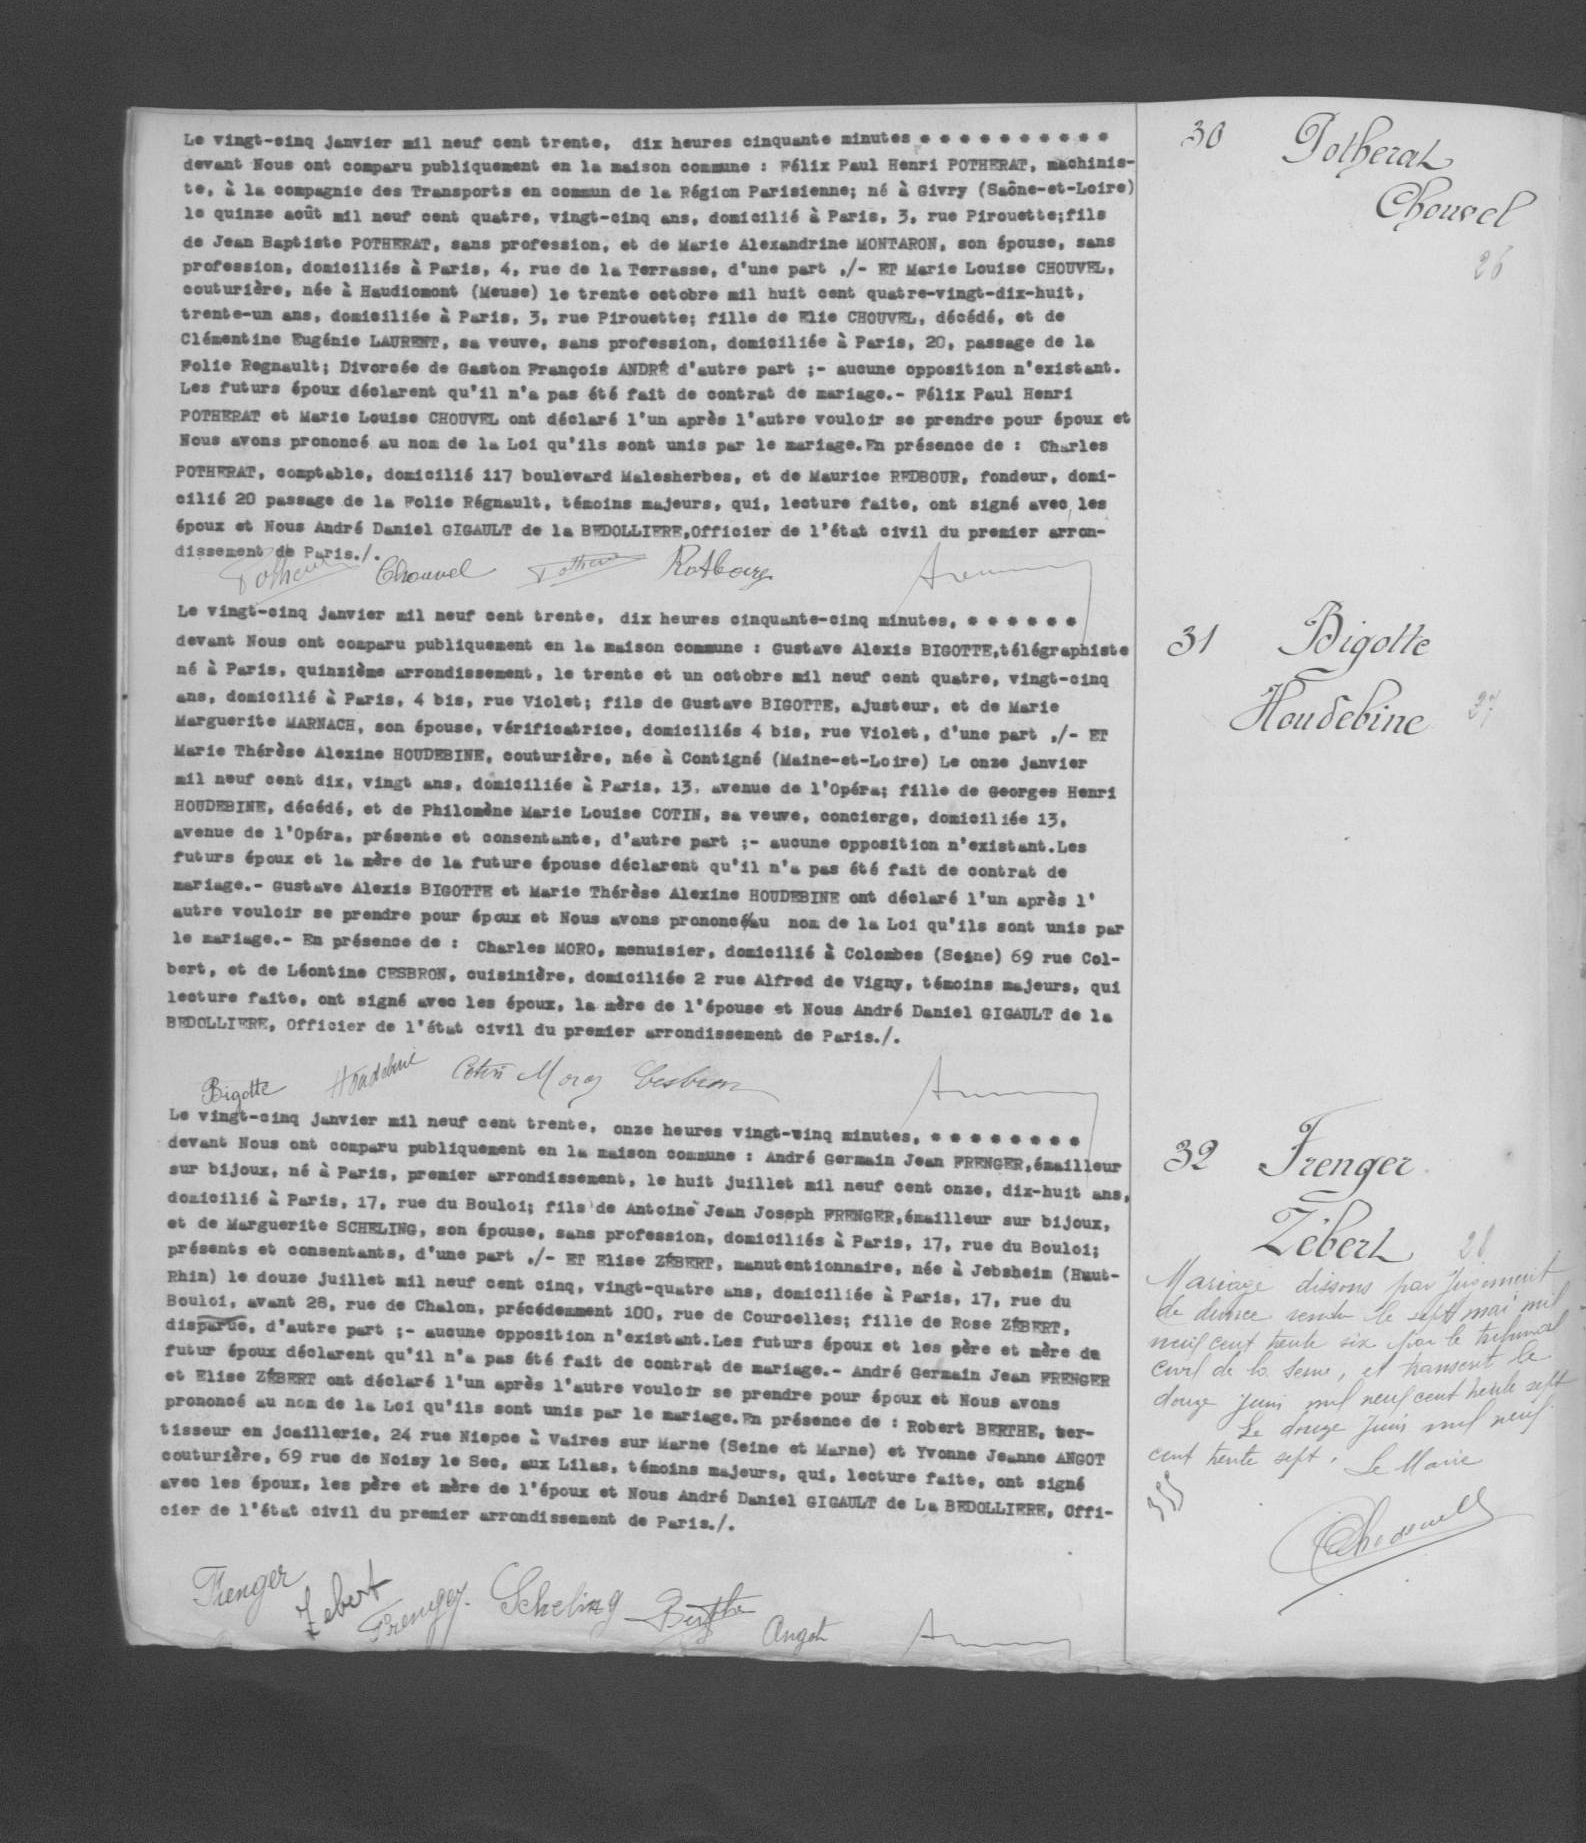Le vingt-cinq janvier mil neuf cent trente, dix heures cinquante minutes ****
devant Nous ont comparu publiquement en la maison commune: Félix Paul Henri POTHERAT, machinis-
te, à la compagnie des Transports en commun de la Région Parisienne; né à Givry (Saône-et-Loire)
le quinze août mil neuf cent quatre, vingt-cinq ans, domicilié à Paris, 3, rue Pirouette; fils
de Jean Baptiste POTHERAT, sans profession, et de Marie Alexandrine MONTARON, son épouse, sans
profession, domiciliés à Paris, 4, rue de la Terrasse, d'une part ./-ET Marie Louise CHOUVEL,
couturière, née à Hauiomont (Meuse) le trente octobre mil huit cent quatre-vingt-dix-huit,
trente-un ans, domiciliée à Paris, 3, rue Pirouette; fille de Elie CHOUVEL, décédé, et de
Clémentine Eugénie LAURENT, sa veuve, sans profession, domiciliée à Paris, 20, passage de la
Folie Regnault; Divorcée de Gaston François ANDRE d'autre part ;-aucune opposition n'existant.
Les futurs époux déclarent qu'il n'a pas été fait de contrat de mariage.-Félix Paul Henri
POTHERAT et Marie Louise CHOUVEL ont déclaré l'un après l'autre vouloir se prendre pour époux et
Nous avons prononcé au nom de la Loi qu'ils sont unis par le mariage. En présence de: Charles
POTHERAT, comptable, domicilié 117 boulevard Malesherbes, et de Maurice REDBOUR, fondeur, domi-
cilié 20 passage de la Folie Régnault, témoins majeurs, qui, lecture faite, ont signé avec les
époux et Nous André Daniel GIGAULT de la BEDOLLIERE, Officier de l'état civil du premier arron-
dissement de Paris ./.
Le vingt-cinq janvier mil neuf cent trente, dix heures cinquante-cinq minutes, ****
devant Nous ont comparu publiquement en la maison commune: Gustave Alexis BIGOTTE, télégraphiste
né à Paris, quinzième arrondissement, le trente et un octobre mil neuf cent quatre, vingt-cinq
ans, domicilié à Paris, 4 bis, rue Violet; fils de Gustave BIGOTTE, ajusteur, et de Marie
Marguerite MARNACE, son épouse, vérificatrice, domiciliés 4 bis, rue Violet, d'une part ./-ET
Marie Thérèse Alexine HOUDEBINE, couturière, née à Contigné (Maine-et-Loire) Le onze janvier
mil neuf cent dix, vingt ans, domiciliée à Paris, 13, avenue de l'Opéra; fille de Georges Henri
HOUDEBINE, décédé, et de Philomène Marie Louise COTIN, sa veuve, concierge, domiciliée 13,
avenue de l'Opéra, présente et consentante, d'autre part ;-aucune opposition n'existant Les
futurs époux et la mère de la future épouse déclarent qu'il n'a pas été fait de contrat de
mariage .-Gustave Alexis BIGOTTE et Marie Thérèse Alexine HOUDEBINE ont déclaré l'un après l'
autre vouloir se prendre pour époux et Nous avons prononcé au nom de la Loi qu'ils sont unis par
le mariage .-En présence de: Charles MORO, menuisier, domicilié à Colombes (Seine) 69 rue Col-
bert, et de Léontine CESBRON cuisinière, domiciliée 2 rue Alfred de Vigny, témoins majeurs, qui
lecture faite, ont signé avec les époux, la mère de l'épouse et Nous André Daniel GIGAULT de la
BEDOLLIERE, Officier de l'état civil du premier arrondissement de Paris ./.
Le vingt-cinq janvier mil neuf cent trente, onze heures vingt-cinq minutes, ****
devant Nous ont comparu publiquement en la maison commune: André Germain Jean FRENGER, émailleur
sur bijoux, né à Paris, premier arrondissement, le huit juillet mil neuf cent onze, dix-huit ans,
domicilié à Paris, 17, rue du Bouloi; fils de Antoine Jean Joseph FRENGER, émailleur sur biijoux,
et de Marguerite SCHELING, son épouse, sans profession, domiciliés à Paris, 17, rue du Bouloi;
présents et consentants, d'une part ./-et Elise ZEBERT, manutentionnaire, née à Jebaheim (Haut-
Rhin) le douze juillet mil neuf cent cinq, vingt-quatre ans, domiciliée à Paris, 17, rue du
Bouloi, avant 26, rue de Chalon précedemment 100, rue de Courcelles; fille de Rose ZEBERT,
disparue, d'autre part ;-aucune opposition n'existant. Les futurs époux et les père et mère de
futur époux déclarent qu'il n'a pas été fait de contrat de mariage. André Germain Jean FRENGER
et Eise ZEBERT ont déclaré l'un après l'autre vouloir se prendre pour époux et Nous avons
prononcé au nom de la Loi qu'ils sont unis par le mariage. En présence de: Robert BERTHE, ver-
nisseur en joiaillerie, 24 rue Nipoe à Vaires sur Marne (Seine et Marne) et Yvonne Jeanne ANGOT
couturière, 69 rue de Noisy le Sec, aux Lilas, témoins majeurs, qui, lecture faite, ont signé
avec les époux, les père et mère de l'époux et Nous André Daniel GIGAULT de la BEDOLLIERE, Offi-
cier de l'état civil du premier arrondissement de Paris ./.
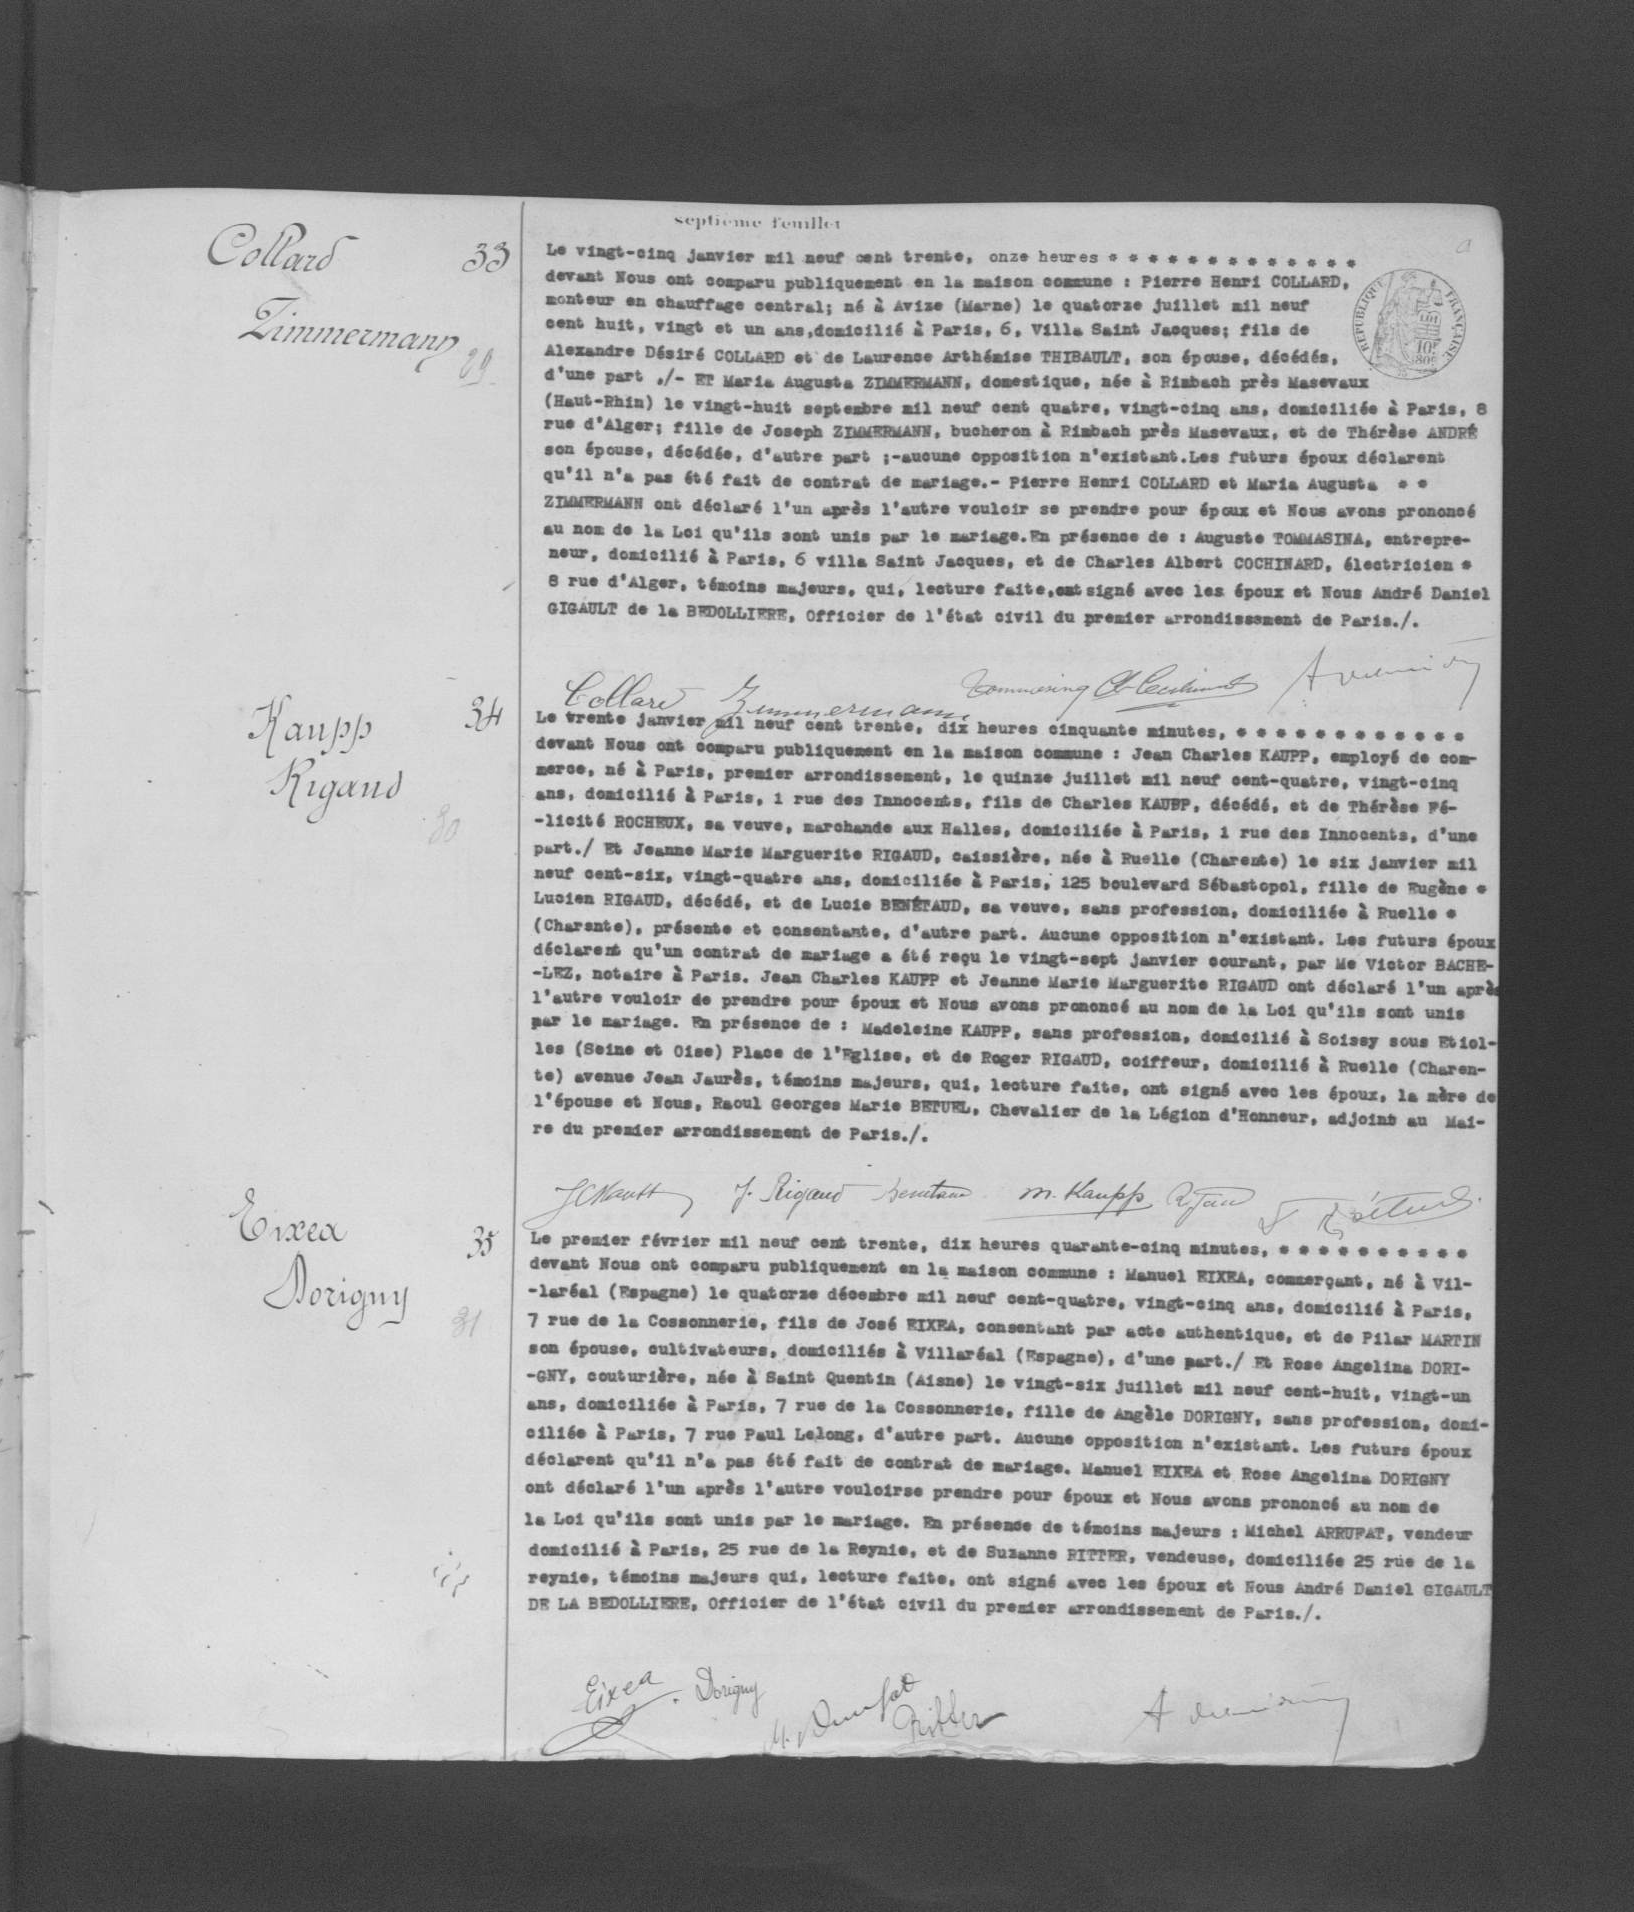Le vingt-cinq janvier mil neuf cent trente, onze heures ****
devant Nous ont comparu publiquement en la maison commune: Pierre Henri COLLARD,
monteur en chauffage central; né à Avize (Marne) le quatorze juillet mil neuf
cent huit, vingt et un ans, domicilié à Paris, 6, Ville Saint-Jacques; fils de
Alexandre Désiré COLLARD et de Laurence Arthémise THIBAULT, son épouse, décédés,
d'une part ./-ET Maria Auguste ZIMMERMANN, domestique, née à Rimbach près Masevaux
(Haut-Rhin) le vingt-huit septembre mil neuf cent quatre, vingt-cinq ans, domiciliée à Paris, 8
rue d'Alger; fille de Joseph ZIMMERMANN, bucheron à Rimbach près Masevaux, et de Thérèse ANDRE
son épouse, décédée, d'autre part ;-aucune opposition n'existant. Les futurs époux déclarent
qu'il n'a pas été fait de contrat de mariage .-Pierre Henri COLLARD et Maria Auguste * *
ZIMMERMANN ont déclaré l'un après l'autre vouloir se prendre pour époux et Nous avons prononcé
au nom de la Loi qu'ils sont unis par le mariage. En présence de: Auguste TOMMASINA, entrepre-
neur, domicilié à Paris, 6 villa Saint Jacques, et de Charles Albert COCHINARD, électricien *
8 rue d'Alger, témoins majeurs, qui, lecture faite, ont signé avec les époux et Nous André Daniel
GIGAULT de la BEDOLLIERE, Officier de l'état civil du premier arrondisement de Paris ./
Le trente janvier mil neuf cent trente, dix heures cinquante minutes, ****
devant Nous ont comparu publiquement en la maison commune: Jean Charles KAUPP, employé de com-
merce, né à Paris, premier arrondissement, le quinze juillet mil neuf cent-quatre, vingt-cinq
ans, domicilié à Paris, 1 rue des Innocents, fils de Charles KAUPP, décédé, et de Thérèse Fé-
-licité ROCHEUX, sa veuve, marchande aux Halles, domiciliée à Paris, 1 rue des Innocents, d'une
part ./ ET Jeanne Marie Marguerite RIGAUD, caissière, née à Ruelle (Charente) le six janvier mil
neuf cent-six, vingt-quatre ans, domiciliée à Paris, 125 boulevard Sébastopol, fille de Eugène *
Lucie RIGAUD, décédé, et de Lucie BENETAUD, sa veuve, sans profession, domiciliée à Ruelle *
(Charente), présente et consentante, d'autre part. Aucune opposition n'existant. Les futurs époux
déclarent qu'un contrat de mariage a été reçu le vingt-sept janvier courant, par Me Victor BACHE-
-LEZ, notaire à Paris. Jean Charles KAUPP et Jeanne Marie Marguerite RIGAUD ont déclaré l'un après
l'autre vouloir se prendre pour époux et Nous avons prononcé au nom de la loi qu'ils unis
par le mariage. En présence de: Madeleine KAUPP, sans profession, domicilié à Soisey sous Etiel-
les (Seine et Oise) Place de l'Eglise, et de Roger RIGAUD, coiffeur, domicilié à Ruelle (Charen-
te) avenue Jean Jaurès, témoins majeurs, qui, lecture faite, ont signé avec les époux, la mère de
l'épouse et Nous, Raoul Georges Marie BETUEL, Chevalier de la Légion d'Honneur, adjoints au Mai-
re du premier arrondissement de Paris ./.
Le premier février mil neuf cent trente, dix heures quarante-cinq minutes, ****
devant Nous ont comparu publiquement en la maison commune: Manuel EIXEA, commerçant, né à Vil
-laréal (Espagne) le quatorze décembre mil neuf cent-quatre, vingt-cinq ans, domicilié à Paris,
7 rue de la Cossonerie, fils de José EXEA, consentant par acte authentique, et de Pilar MARTIN
son épouse, cultivateurs, domiciliés à Villaréal (Espagne), d'une part ./ ET Rose Angelina DORI-
-GNY, couturière, née à Saint Quentin (Aisne) le vingt-six juillet mil neuf cent-huit, vingt-un
ans, domiciliée à Paris, 7 rue de la Cossonnerie, fille de Angèle DORIGNY, sans profession, domi-
ciliée à Paris, 7 rue Paul Lelong, d'autre part. Aucune opposition n'existant. Les futurs époux
déclarent qu'il n'a pas été fait de contrat de mariage. Manuel EIXEA et Rose Angelina DORIGNY
ont déclaré l'un après l'autre vouloirse prendre pour époux et Nous avons prononcé au nom
la Loi qu'ils sont unis par le mariage. En présence de témoins majeurs: Michel ARRUPAT, vendeur
domicilié à Paris, 25 rue de la Reynie, et de Suzanne RITTER, vendeuse, domiliée 25 rue de la
reynie, témoins majeur qui, lecture faite, ont signé avec les époux et Nous André Daniel GIGAULT
DE LA BEDOLLIERE, Officier de l'état civil du premier arrondissement de Paris ./.